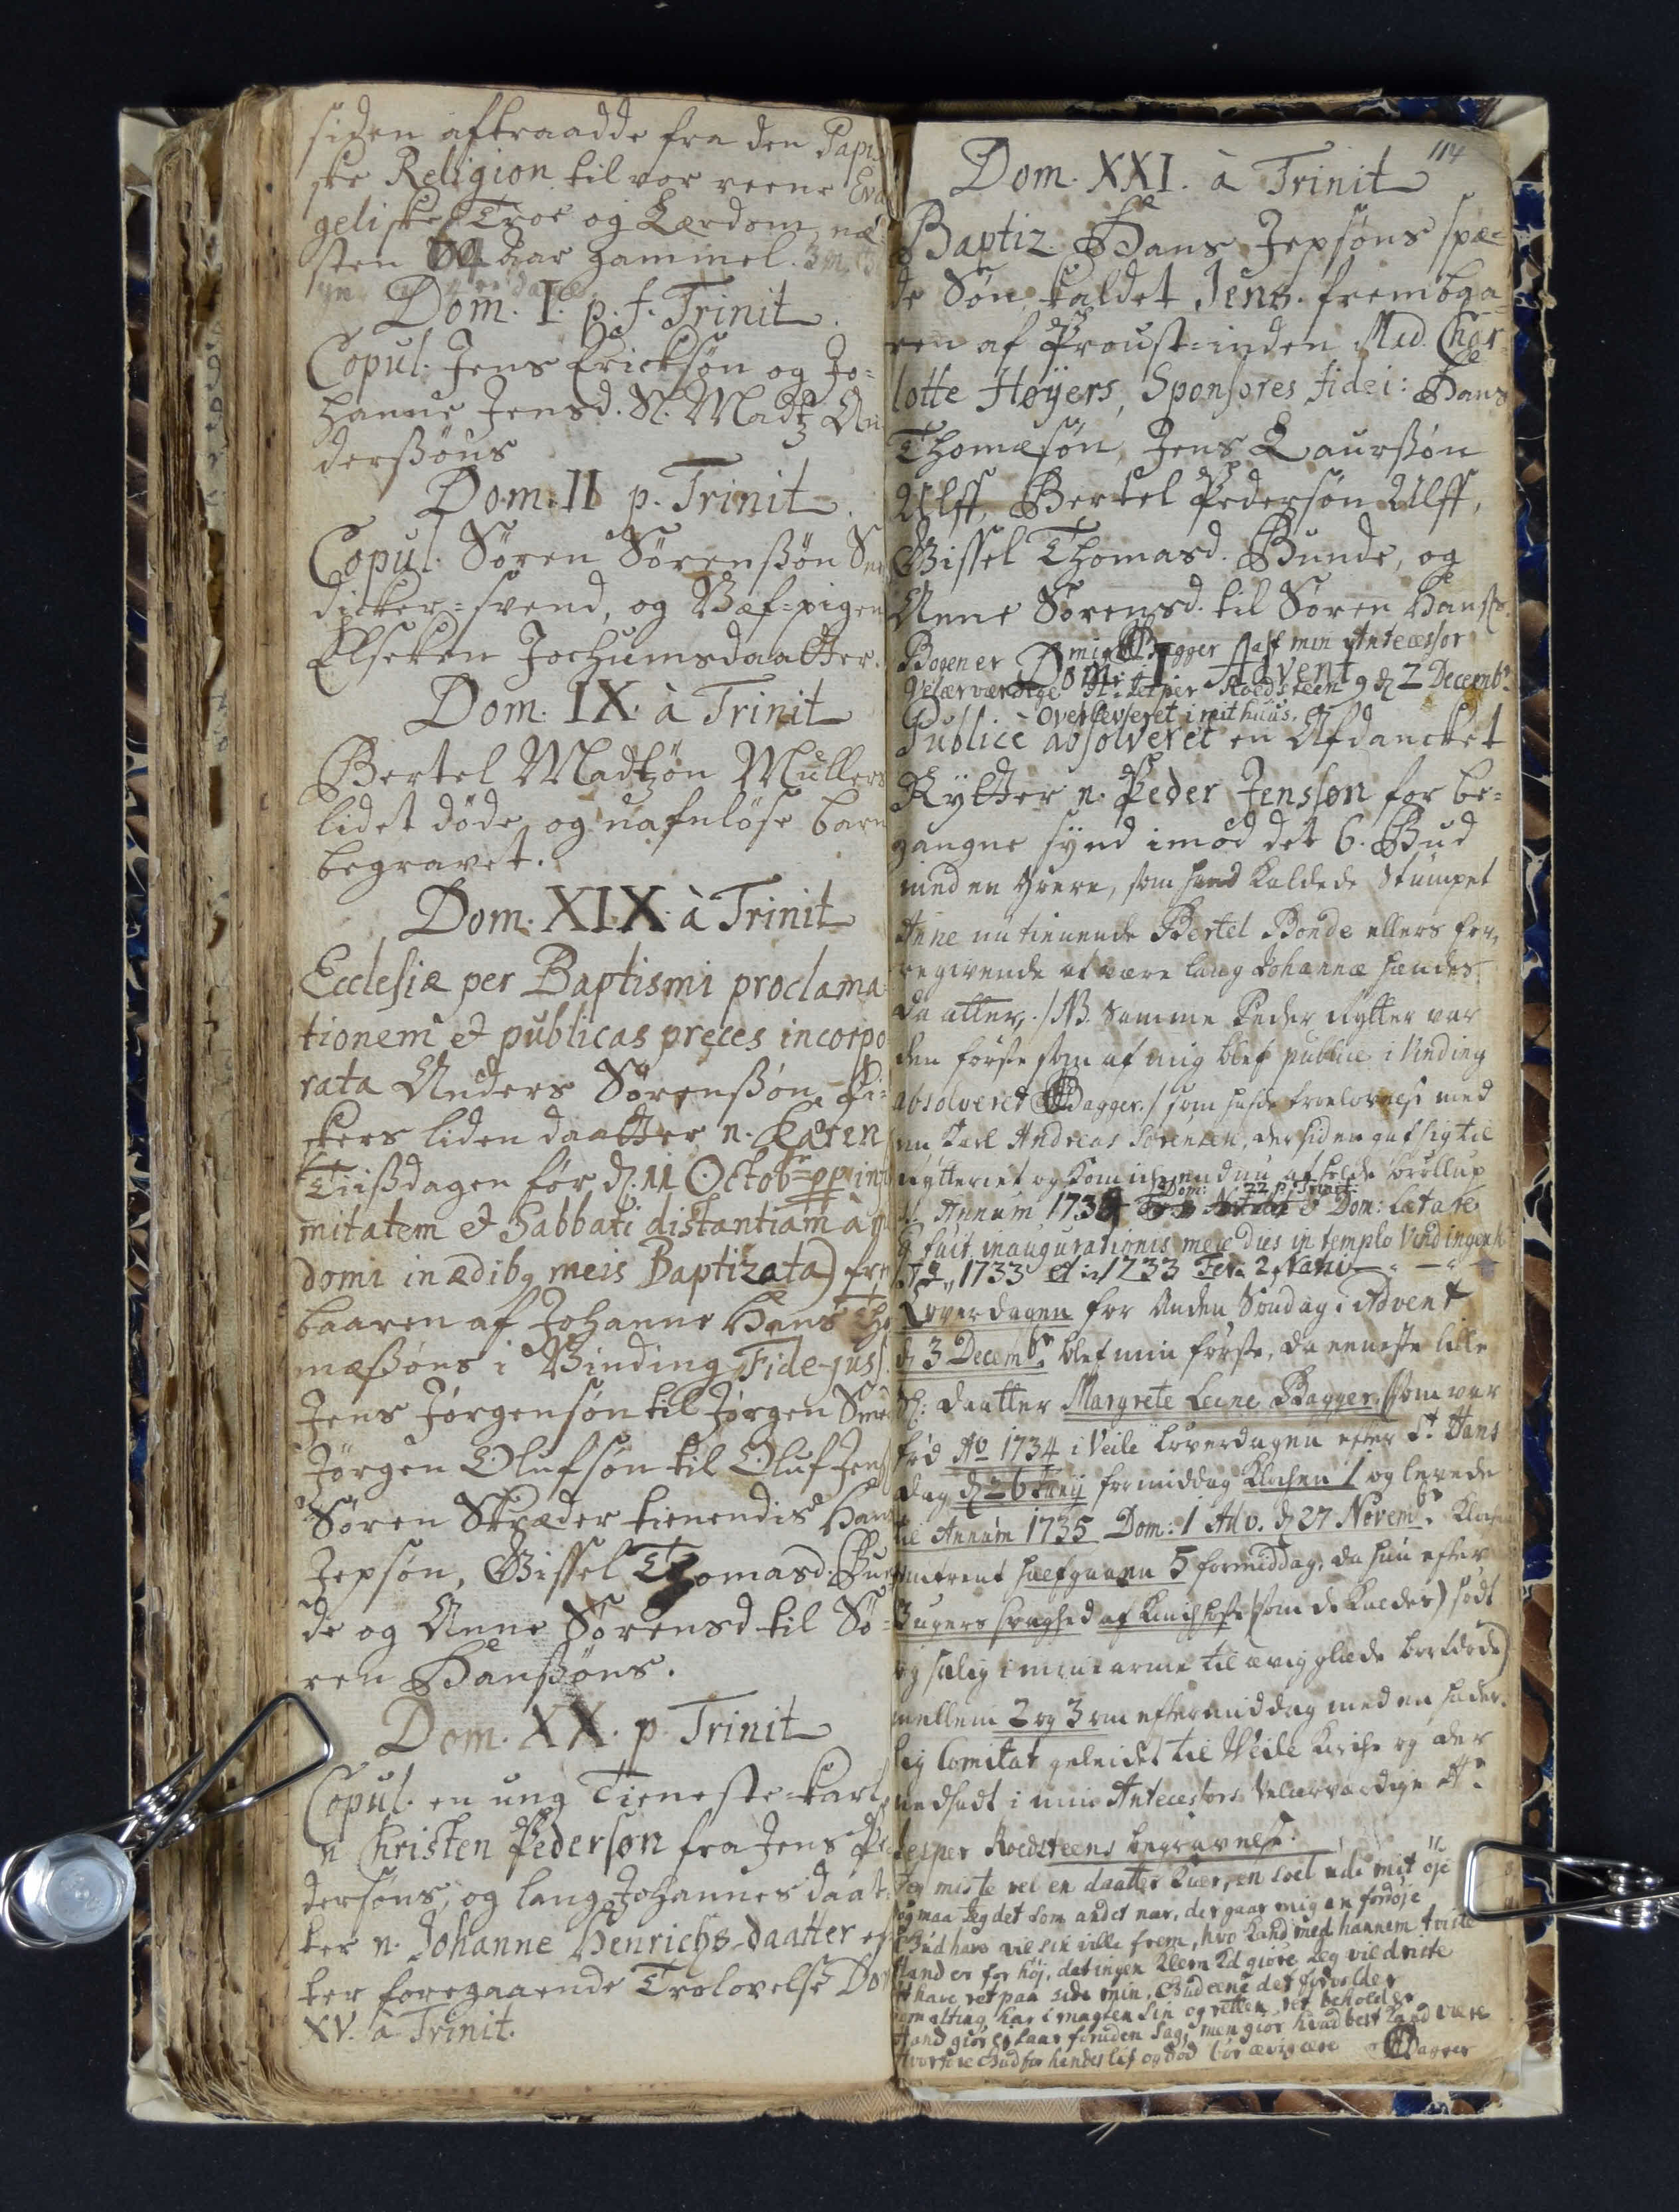siden aftraadde fra den
ske Religion til vor reene Eva  
geliske Troe og Lærdom, næ¬
sten 64 Aar gammel.
Dom. I. p. f. Trinit.
Copul. Jens Erichsøn og Jo¬
hanne Jensd. Sl. Madtz An
derssøns
Dom. II p. Trinit
Copul. Søren Sørenssøn Sne
dicker=svend, og Væf=pigen 
Elseken Jochumsdaatter.
Dom. IX. a Trinit
Bertel Madtzøn Mullers
lidet døde og nafnløse barn
begravet.
Dom. XIX. a Trinit
Ecclesiæ per Baptismi proclama¬
tionem et publicas preces incorpo¬
rata Anders Sørenssøn Fi¬
skers liden daatter n. Karen
(Tiissdagen før d. 11 Octobr pp infi 
mitatem et Sabbati distantiam a m  
domi in ædibq meis Baptizata) fre  
baaren af Johanne Hans Tho  
mæsøns i Vinding. Fide-Juss
Jens Jørgensøn til Jørgen Smed  
Jørgen Olufsøn til Oluf Jens
Søren Skræder tienendis Hans  
Jepsøn, Gissel Thomasd. Bun  
de og Anne Sørensd til Sø¬
ren Hanssøns.
Dom. XX. p. Trinit
Copul. en ung Tieneste=karl
n. Christen Pedersøn fra Jens Pe
dersøns, og lang Johannes daat¬
ter n. Johanne Henrichs-daatter ef
ter foregaaende Trolovelse Dom  
XV. a Trinit.
114
Dom. XXI. a Trinit
Baptiz. Hans Jepsøns spæ¬
de Søn kaldet Jens. frembaa¬
ren af Poust=inden Mad. Char¬
lotte Høyers, Sponsores fidei: Hans
Thomæsøn, Jens Laurssøn
Ulff, Bertel Pedersøn Ulff,
Gissel Thomasd. Bunde, og
Anne Sørensd. til Søren Hanss
Bogen er mig O Bagger af min Antecessor
Velærværdige Hr Jesper Roedsteen q d 2 Decembr
overleveret i mit huus.
Dom. I Advent.
Publice absolveret en Afdancket
Rytter n. Peder Jensøn for be¬
gangne synd imod det 6 Bud
med en Horre, som hand kaldede Stumpet
Anne nu tienende Bertel Bonde ellers for¬
egivende at være lang Johannæ hændes
daatter, / NB. Samme Peder Rytter var
den første som af mig blef publice i Vinding
absolveret O Bagger. / som hafde troelovelse med
een Karl Andreas Sørensen, der siden gaf sig til
##ytteriet, og kom iche endnu at holde brøllup
Dom: 22 p. Trinit:
## Annum 173## ## ## et Dom: Lætare
q fait inaugurationis me## dies in templo Vindinge##
##, 1733 et in 1733 Fer. 2 Nativ.
Løverdagen for Anden Søndag i Advent
d 3 Decembr blef min første, da eeneste lille
Sl: daatter Margrete Leene Bagger (som var
fød Ao 1734 i Veile Løverdagen efter St Hans
dag d 26 Junij formiddag Klochen 1 og levede
til Annum 1735 Dom: 1 Adv. d 27 Novembr Klocken
omtrent halfgaaen 5 formiddag, da hun efter
3 ugers svaghed af Kinchhoste (som de kaldes) sødt
og salig i mine arme til ævig glæde bortdøde)
mellem 2 og 3 om eftermiddagen med en fader¬
lig Comitat geleidet til Weile Kirche og der
nedsadt i min Antecessors Velærværdige Hr
Jesper Roedsteens begravelse.
Jeg miste vel en daatter Kiær, en soel udi mit øje
og maa jeg det som andet nær der gaar mig an fordøje
Gud have vil sin ville frem, hvo kand med hennem tviste
hand er for høj, det ingen Kloen kd giøre Jeg vil driste
at have ret paa side min, Gud eene det forvolder
som alting, har i magten sin og retten ret beholder
Hand giør ei hans foruden Sag, men giør hvad best kand være
Hvorfore Gud for hendes lif og død bør ævig ære. OHBagger.
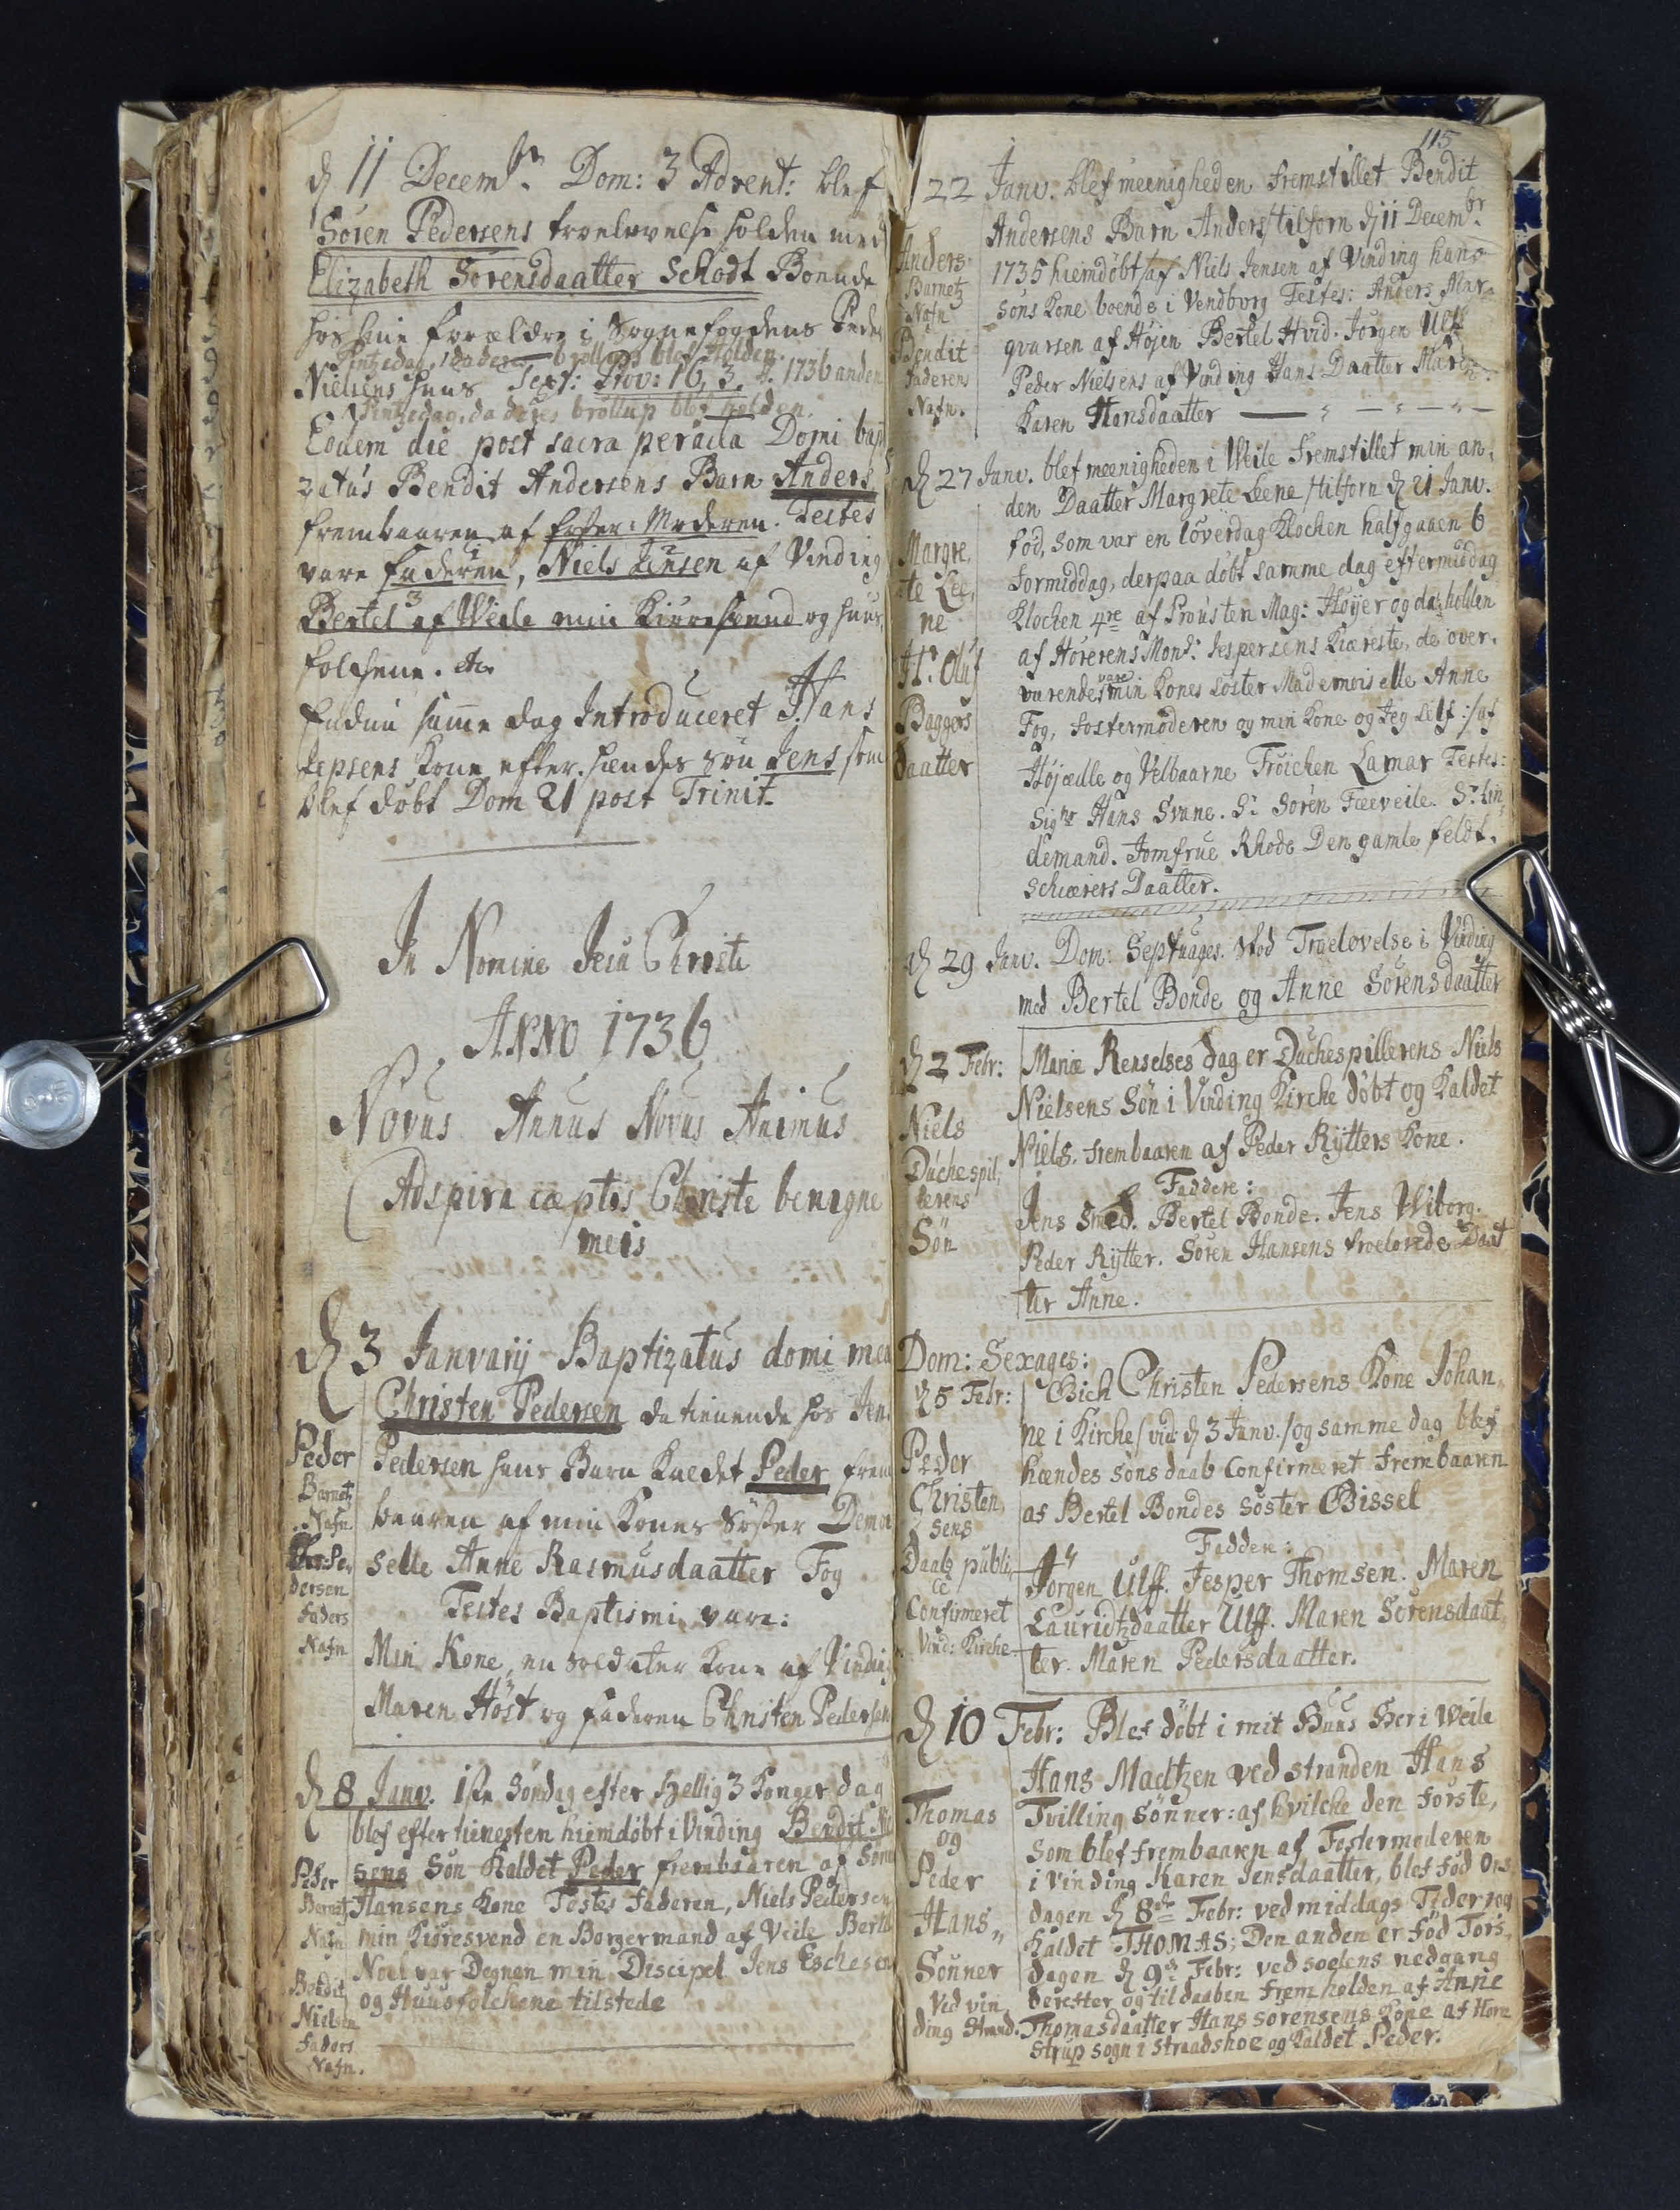d 11 decembr Dom: 3 Advent: blef
Søren Pedersens trolovelse holden med
Elisabeth Sørensdaatter Schodt Boende
hos sine forældre i Sognefogdens Peder